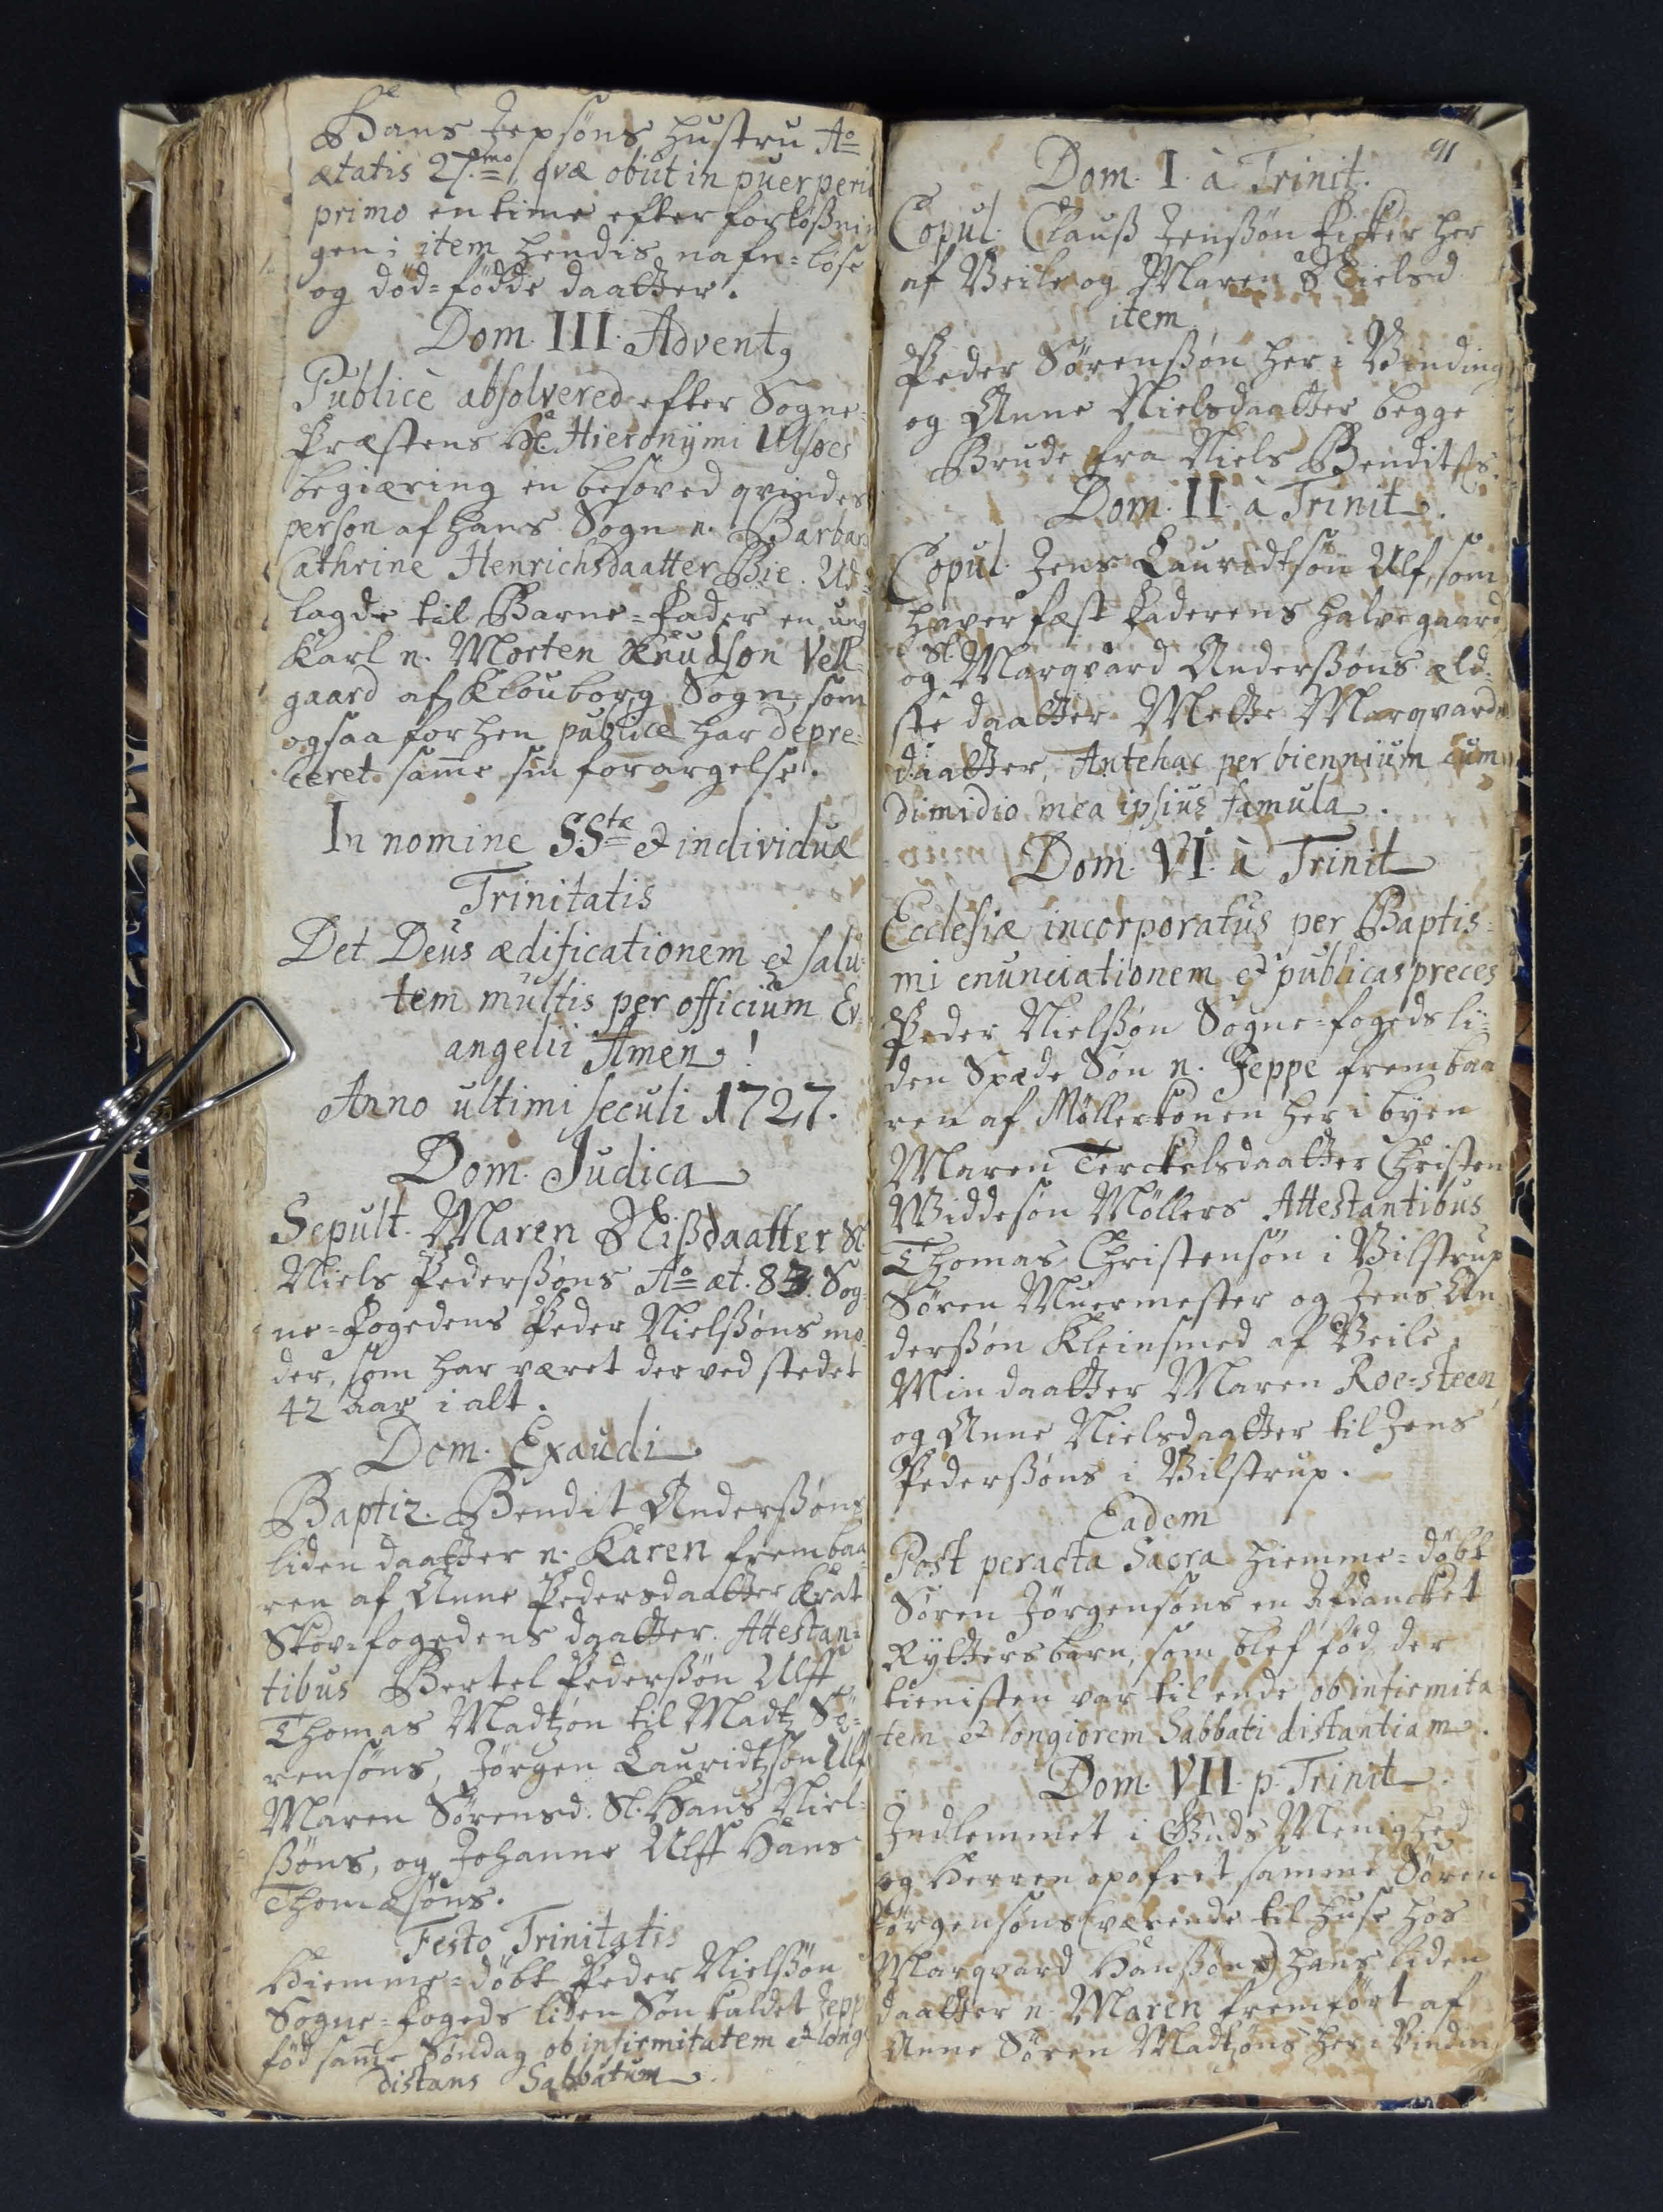Hans Jepsøns hustru Ao
ætatis 27mo qvæ obiit in puerperi 
primo en time efter forløsnin
gen i item hendis nafn=løse
og død=fødde daatter.
Dom. III. Adventq
Publice absolvered efter Sogne¬
Præstens Hl Hieronymi Ulsøes
begiæring en besoved qvindes
person af hans Sogn n. Barbar 
Cathrine Henrichsdaatter Bie. Ud¬
lagde til Barne=Fader en ung
Karl n. Morten Knudsøn Vell¬
gaard af Klouborg Sogn, som
ogsaa for hen publice har depre¬
ceret samme sin forargelse.
In nimine S.Stæ et individuæ
Trinitatis
Det Deus ædificationem et sali¬
tem multis per officium Ev
angelii Amen
Anno ultimi seculi 1727
Dom. Judica
Sepult. Maren Nissdaatter Sl.
Niels Pederssøns Ao æt. 83, Sog¬
ne=Fogedens Peder Nielsøns mo¬
der, som har været der ved stedet 
42 Aar i alt.
Dom. Exaudi.
Baptiz. Bendit Anderssøns
liden daatter n. Karen, frembaa¬
ren af Anne Pedersdaatter Krat
Skov=fogedens daatter. Attestan¬
tibus Bertel Pederssøn Ulff
Thomas Madtzøn til Madtz Sø¬
rensøns, Jørgen Lauridtzøn Ulf 
Maren Sørensd. Sl. Hans Niel¬
ssøns, og Johanne Ulff Hans
Thomæsøns.
Festo Trinitatis
Hiemmedøbt Peder Nielssøn
Sogne=fogeds liden Søn kaldet Jepp 
fød samme Søndag ob infirmitatem et long
distans Sabbatum
91
Dom. I. a Trin
Copul. Clauss Jenssøn Fisker her
af Veile og Maren Nielsd
item
Peder Sørenssøn her i Vinding
og Anne Nielsdaatter, begge
Brude fra Niels Benditss
Dom. II. a Trinit
Copul. Jens Lauridtzøn Ulf, som
haver fæst Faderens halve gaard
Sl.
og Marqvard Anderssøns æld¬
ste daatter Mette Marqvards
daatter, Antehac per biennium cum
dimidio mea ipsius famula.
Dom. VI. a Trin
Ecclesiæ incorporatus per Baptis¬
mi enuneiationem et publicas preces
Peder Nielssøn Sogne=Fogeds li¬
den Spæde Søn n. Jeppe frembaa
ren af Møller=konen her i byen
Maren Terckelsdaatter Christen
Widdesøn Møllers Attestantibus
Thomas Christensøn i Vilstrup
Søren Muermester og Jens An
derssøn Kleinsmed af Veile
Min daatter Maren Roe=steen
og Anne Nielsdaatter til Jens
Pederssøns i Vilstrup.
Eadem
Post peracta Sacra hiemme=døbt
Søren Jørgensøns en Afdancket
Rytters barn som blef fød der
tienisten var til ende ob infirmita
tem et longiorem Sabbati distantiam
Dom. VII. p. Trin.
Indlemmet i Guds Menighed
og Herren opofret samme Søren
Jørgensøns (værende til huse hos
Marqvard Hanssøn ##) hans liden
daatter n. Maren, fremført af
Anne Søren Madtzøns her i Vinding
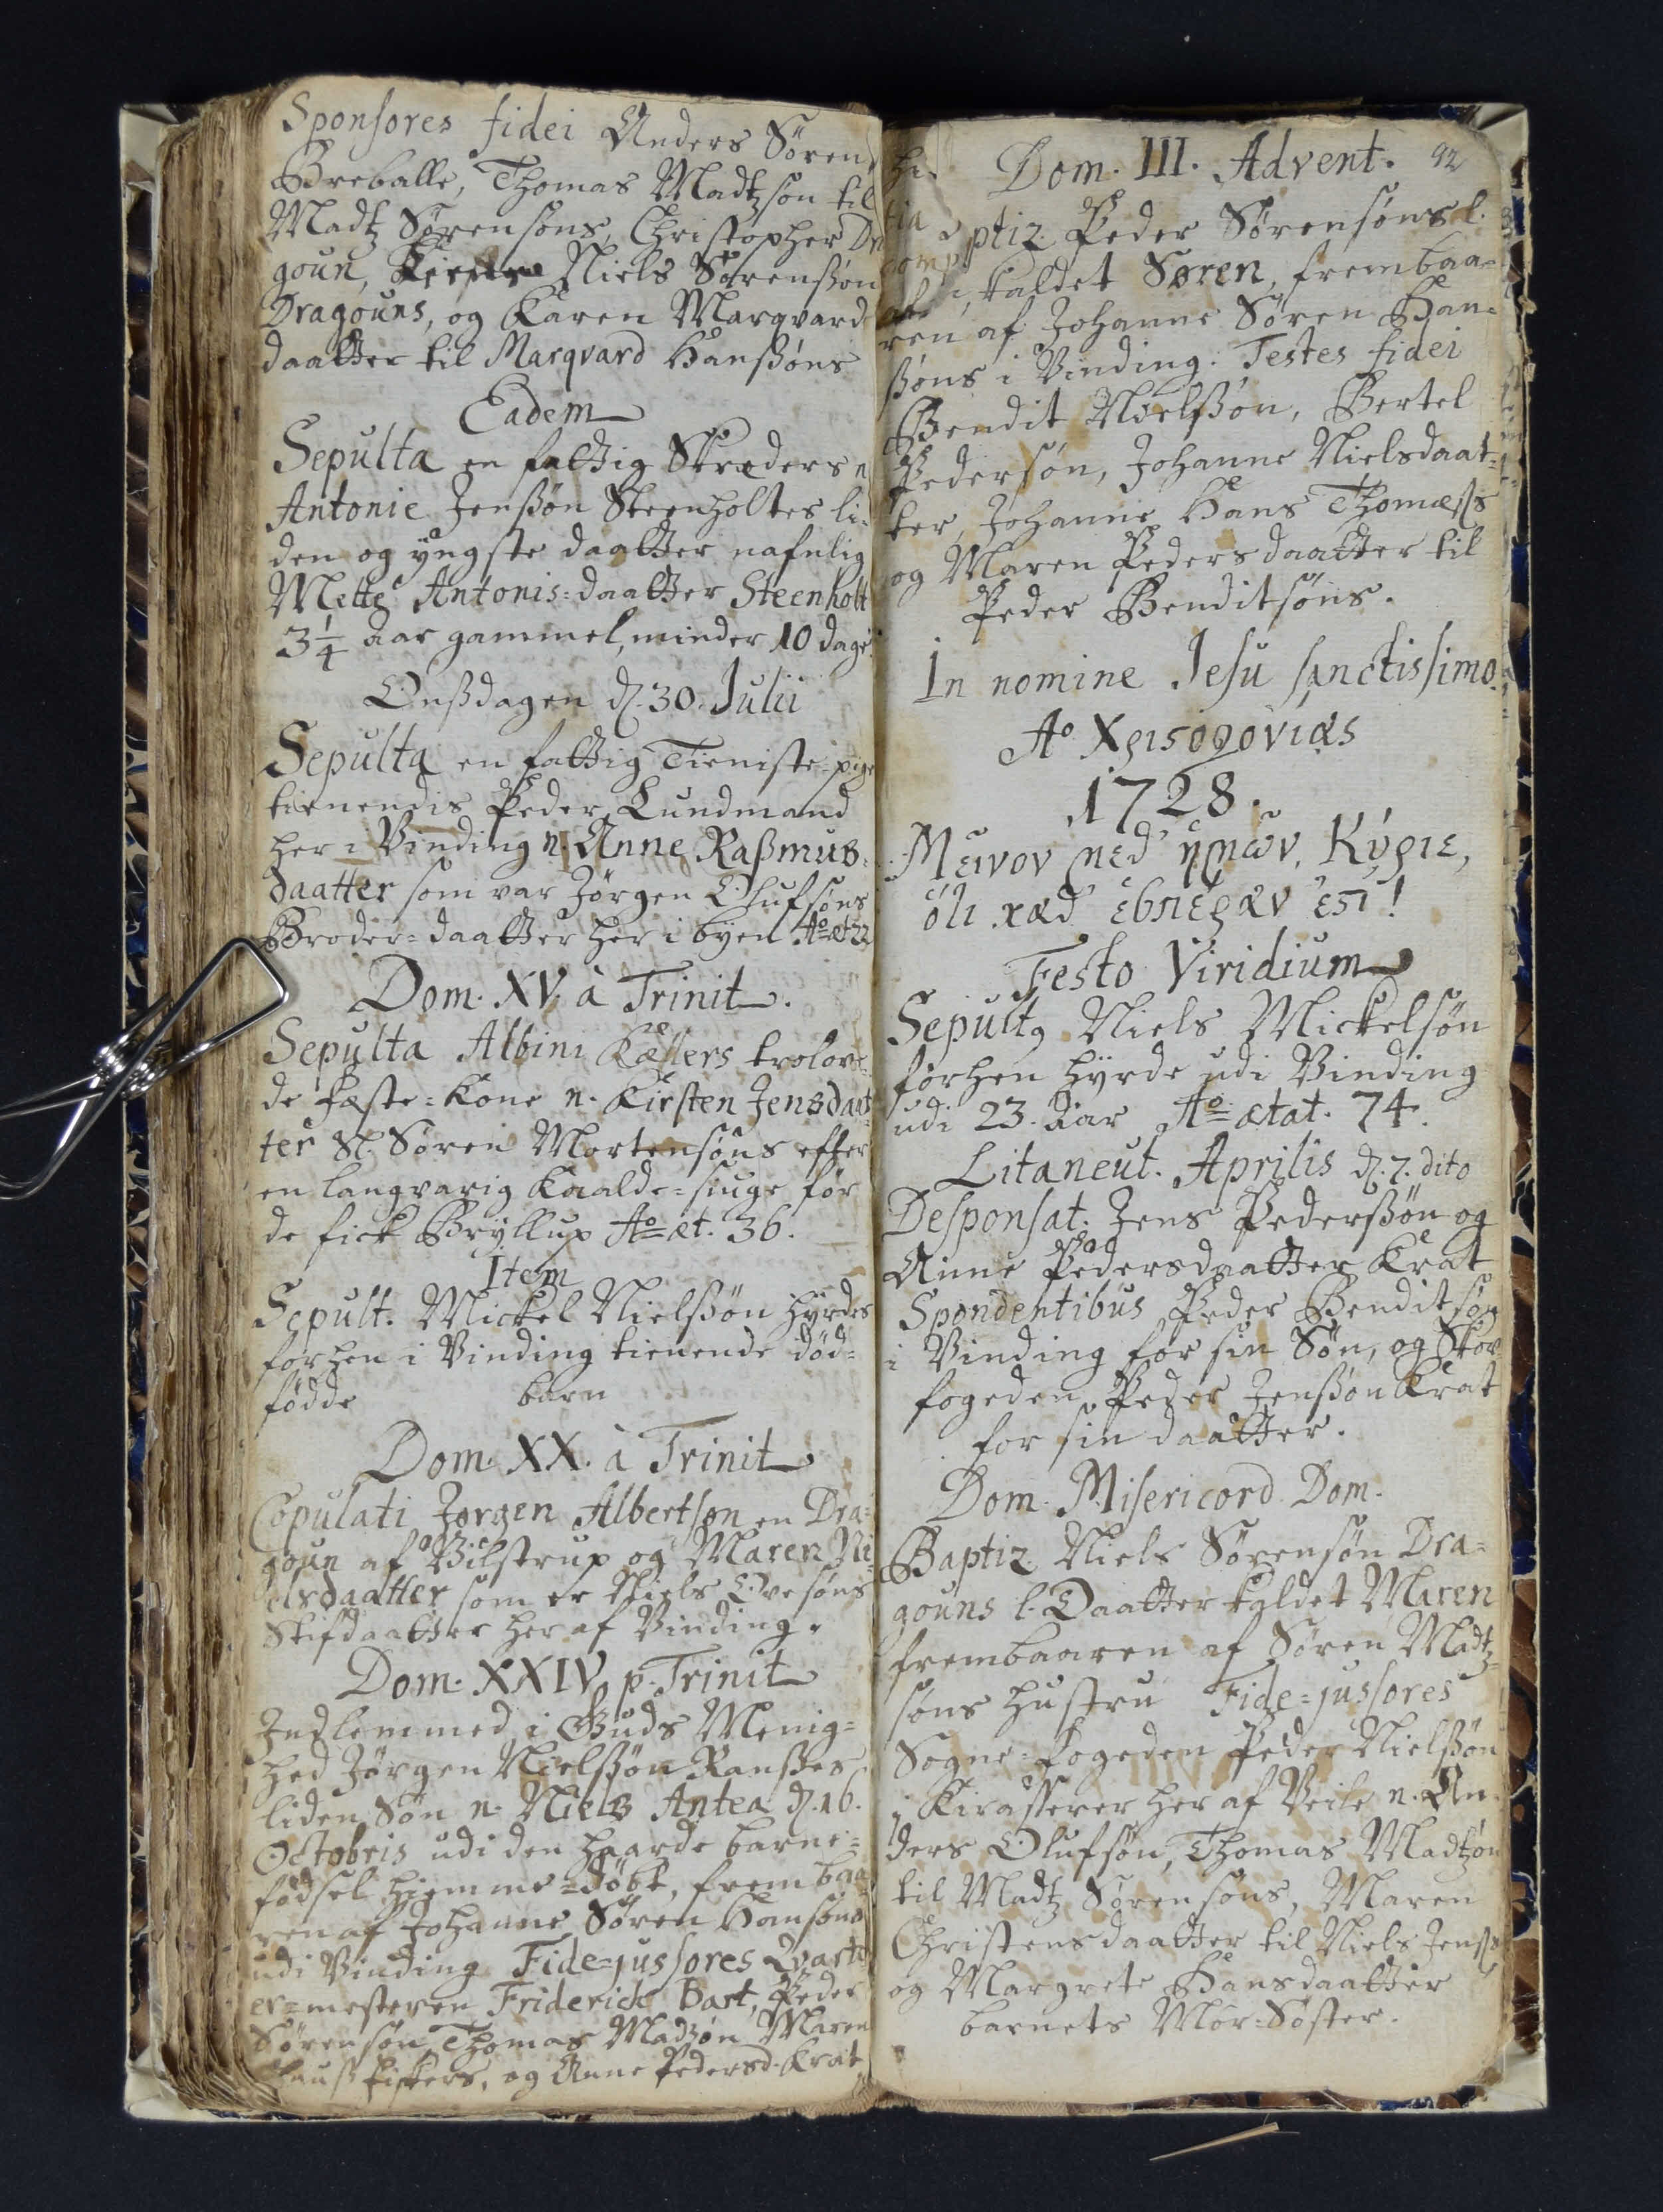Sponsores fidei Anders Søren 
Breballe, Thomas Madtzsøn til
Madtz Sørensøns, Christopher Dr 
goun, Kirsten Niels Sørenssøn
Dragouns, og Karen Marqvard 
daatter til Marqvard Hanssøns.
Eadem
Sepulta en fattig Skræders n
Antonie Jenssøn Steenholtes li¬
den og yngste daatter nafnlig
Mette Antonis=daatter Steenholt
3¼ Aar gammel, minder 10 dage.
Onssdagen d. 30. Julii
Sepulta en fattig Tieniste-pige
tienendis Peder Lundmand
her i Vinding n. Anne Rassmus¬
daatter som var Jørgen Olufsøns
Broder=daatter her i byen Ao æt 22
Dom. XV a Trinit.
Sepulta Albini Kællers trolove¬
de Fæste=Kone n. Kirsten Jensdaat¬
ter Sl. Søren Mortensøns efter
en langvarig Kaalde=siuge, før
de fick Bryllup Ao æt. 36.
Item
Sepult. Mickel Nielssøn hyrdes
forhen i Vinding tienende død¬
fødde
barn
Dom. XX. a Trinit.
Copulati Jørgen Albertsøn en Dra¬
goun af Vilstrup og Maren Ni¬
elsdaatter som er Niels Ovesøns
Stifdaatter her af Vinding.
Dom. XXIV p. Trinit
Indlemmed i Guds Menig¬
hed Jørgen Nielssøn Ransses
liden Søn n. Niels Antea d. 16.
Octobris udi den haarde barne¬
fødsel hiemme=døbt, frembaa¬
ren af Johanne Søren Hansøns
udi Vinding Fide=jussores Qvarte¬ 
er=mesteren Friderich Bart. Peder
Sørensøn, Thomas Madzøn Maren
Clauss Fiskers, og Anne Pedersd. Krat
92
Dom. III. Advent.
ptiz. Peder Sørensøns l.
kaldet Søren, frembaa¬
ren af Johanne Søren Han¬
ssøns i Vinding. Testes fidei
Bendit Nielssøn, Bertel
Pedersøn, Johanne Nielsdaat¬
ter. Johanne Hans Thomæss
og Maren Pedersdaatter til
Peder Benditsøns.
In nomine Jesu sanctissimo
Ao X##vias
1728
Meivov ned nmav, Kvqie
oli xæd eb## e##
Festo Viridium
Sepultq Niels Mickelsøn
forhen hyrde udi Vinding
udi 23. Aar Ao ætat. 74.
Litaneut Aprilis d. 7. dito
Desponsat. Jens Pederssøn og
Anne Pedersdaatter Krat
Spondentibus Peder Benditsøn
i Vinding for sin Søn, og Skov¬
fogeden Peder Jenssøn Krat
for sin daatter.
Dom. Misericord. Dom.
Baptiz. Niels Sørensøn Dra¬
gouns l. Daatter kaldet Maren
frembaaren af Søren Madtz¬
søns hustru Fide=jussores
Sogne=Fogeden Peder Nielssøn.
1 Kirasserer her af Veile n. An¬
ders Olufsøn, Thomas Madtzøn
til Madtz Sørensøns, Maren
Christensdaatter til Niels Jenss
og Margrete Hansdaatter 
barnets Mor=Søster.